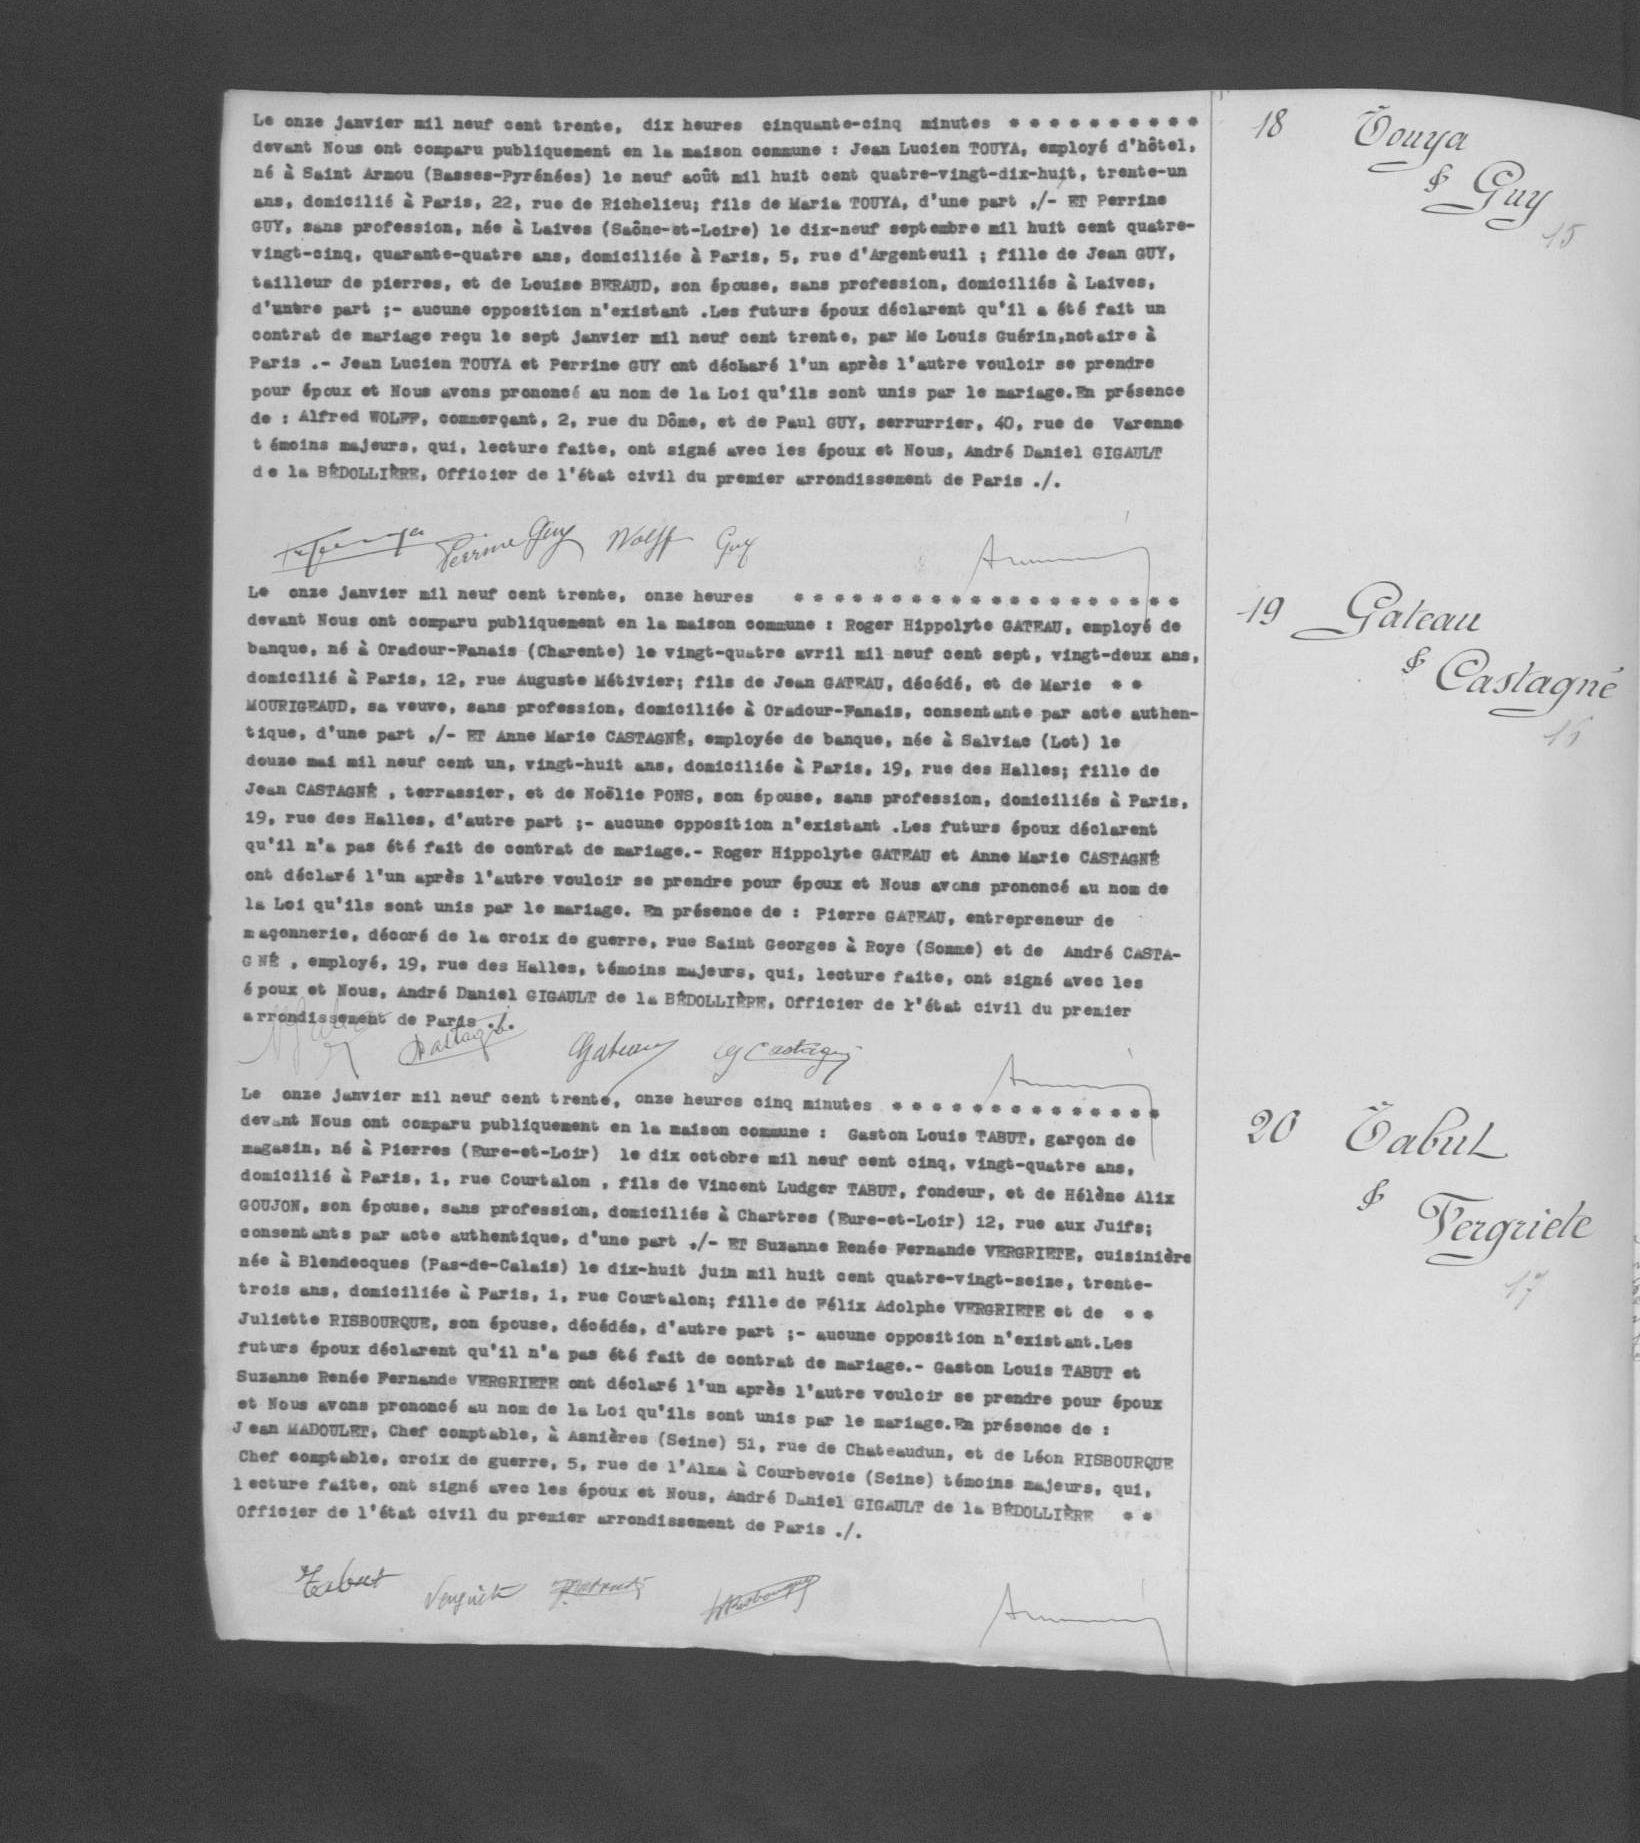Le onze janvier mil neuf cent trente, dix heures cinquante-cinq minutes ****
devant Nous ont comparu publiquement en la maison commune: Jean Lucien TOUYA, employé d'hôtel,
né à Saint Armou (Basses-Pyrénées) le neuf août mil huit cent quatre-vingt-dix-huit, trente-un
ans, domicilié à Paris, 22, rue de Richelieu; fils de Marie Touya, d'une part ./-ET Perrine
GUY, sans profession, née à Laives (Saône-et-Loire) le dix-neuf septembre mil huit cent quatre-
vingt-cinq, quarante-quatre ans, domiciliée à Paris, 5, rue d'Argenteuil; fille de Jean GUY,
tailleur de pierres, et de Louise BRRAUD, son épouse, sans profession, domiciliés à Laives,
d'autre part ;-aucune opposition n'existant. Les futurs époux déclarent qu'il a été fait un
contrat de mariage reçu le sept janvier mil neuf cent trente, par Mr Louis Guérin, notaire à
Paris .-Jean Lucien TOUYA et Perrine GUY ont déclaré l'un après l'autre vouloir se prendre
pour époux et Nous avons prononcé au nom de la Loi qu'ils sont unis par le mariage. En présence
de: Alfred WOLFF, commerçant, 2, rue du Dôme, et de Paul GUY, serrurrier, 40, rue de Varenne
témoins majeurs, qui, lecture faite, ont signé avec les époux et nous, André Daniel GIGAULT
de la BEDOLLIERE, Officier de l'état civil du premier arrondissement de Paris ./.
Le onze janvier mil neuf cent trente, onze heures ****
devant Nous ont comparu publiquement en la maison commune: Roger Hippoyte GATEAU, employé de
banque, né à Oradour-Fanais (Charente) le vingt-quatre avril mil neuf cent sept, vingt-deux ans,
domicilié à Paris, 12, rue Auguste Métivier; fils de Jean GATEAU, décédé, et de Marie * *
MOURIGEAUD, sa veuve, sans profession, domiciliée à Oradour-Fanais, consentante par acte authen
tique, d'une part ./-ET Anne Marie CASTAGNE, employée de banque, née à Salviac (Lot) le
douze mai mil neuf cent un, vingt-huit ans, domiciliée à Paris, 19, rue des Halles; fille de
Jean CASTAGNE, terrassier, et de Noëllie PONS, son épouse, sans profession, domiciliés à Paris,
19, rue des Halles, d'autre part ;-aucune opposition n'existant. Les futurs époux déclarent
qu'il n'a pas été fait de contrat de mariage .-Roger Hippolyte GATEAU et Anne Marie CASTAGNE
ont déclaré l'un après l'autre vouloir se prendre pour époux et Nous avons prononcé au nom de
la Loi qu'ils sont unis par le mariage. En présence de: Pierre GATEAU, entepreneur de
maçonnerie, décoré de la croix de guerre, rue Saint Georges à Roye (Somme) et de André CASTA-
GNE, employé, 19, rue des Halles, témoins majeurs qui, lecture faite, ont signé avec les
époux et Nous, André Daniel GIGAULT de la BEDOLLIERE, Officier de l'état civil du premier
arrondissement de Paris ./.
Le onze janvier mil neuf cent trente, onze heures cinq minutes ****
devant Nous ont ont comparu publiquement en la maison commune: Gaston Louis TABUT, garçon de
magasin, né à Pierres (Eure-et-Loir) le dix octobre mil neuf cent cinq, vingt-quatre ans,
domicilié à Paris, 1, rue Courtalon, fils de Vincent Ludger TABUT, fondeur, et de Hélène Alix
GOUJON, son épouse, sans profession, domiciliée à Chartres (Eure-et-Loir), 12, rue aux Juifs;
consentants par acte authentique, d'une part ./-ET Suzanne Renée Ferande VERGRIETE, cuisinière
née à Blendecques (Pas-de-Calais) le dix-huit juin mil huit cent quatre-vingt-seize, trente-
trois ans, domiciliée à Paris, 1, rue Courtalon; fille de Félix Adolphe VERGRIETE et de * *
Juliette RISBOURQUE, son épouse, décédés, d'autre part ;-aucune opposition n'existant. Les
futurs époux déclarent qu'il n'a pas été fait de contrat de mariage .-Gaston Louis TABUT et
Suzanne Renée Fernande VERGRIETE ont déclaré l'un après l'autre vouloir se prendre pour époux
et Nous avons prononcé au nom de la Loi qu'ils sont unis par le mariage. En présence de:
Jean MADOULET, Chef comptable, à Asnières (Seine) 51, rue de Chateaudun, et de Léon RISBOURQUE
Chef comptable, croix de guerre, 5, rue de l'Alsa à Courbevoie (Seine) témoins majeurs, qui,
lecture faite, ont signé avec les époux et Nous, André Daniel GIGAULT de la BEDOLLIERE * *
Officier de l'état civil du premier arrondissement de Paris ./.
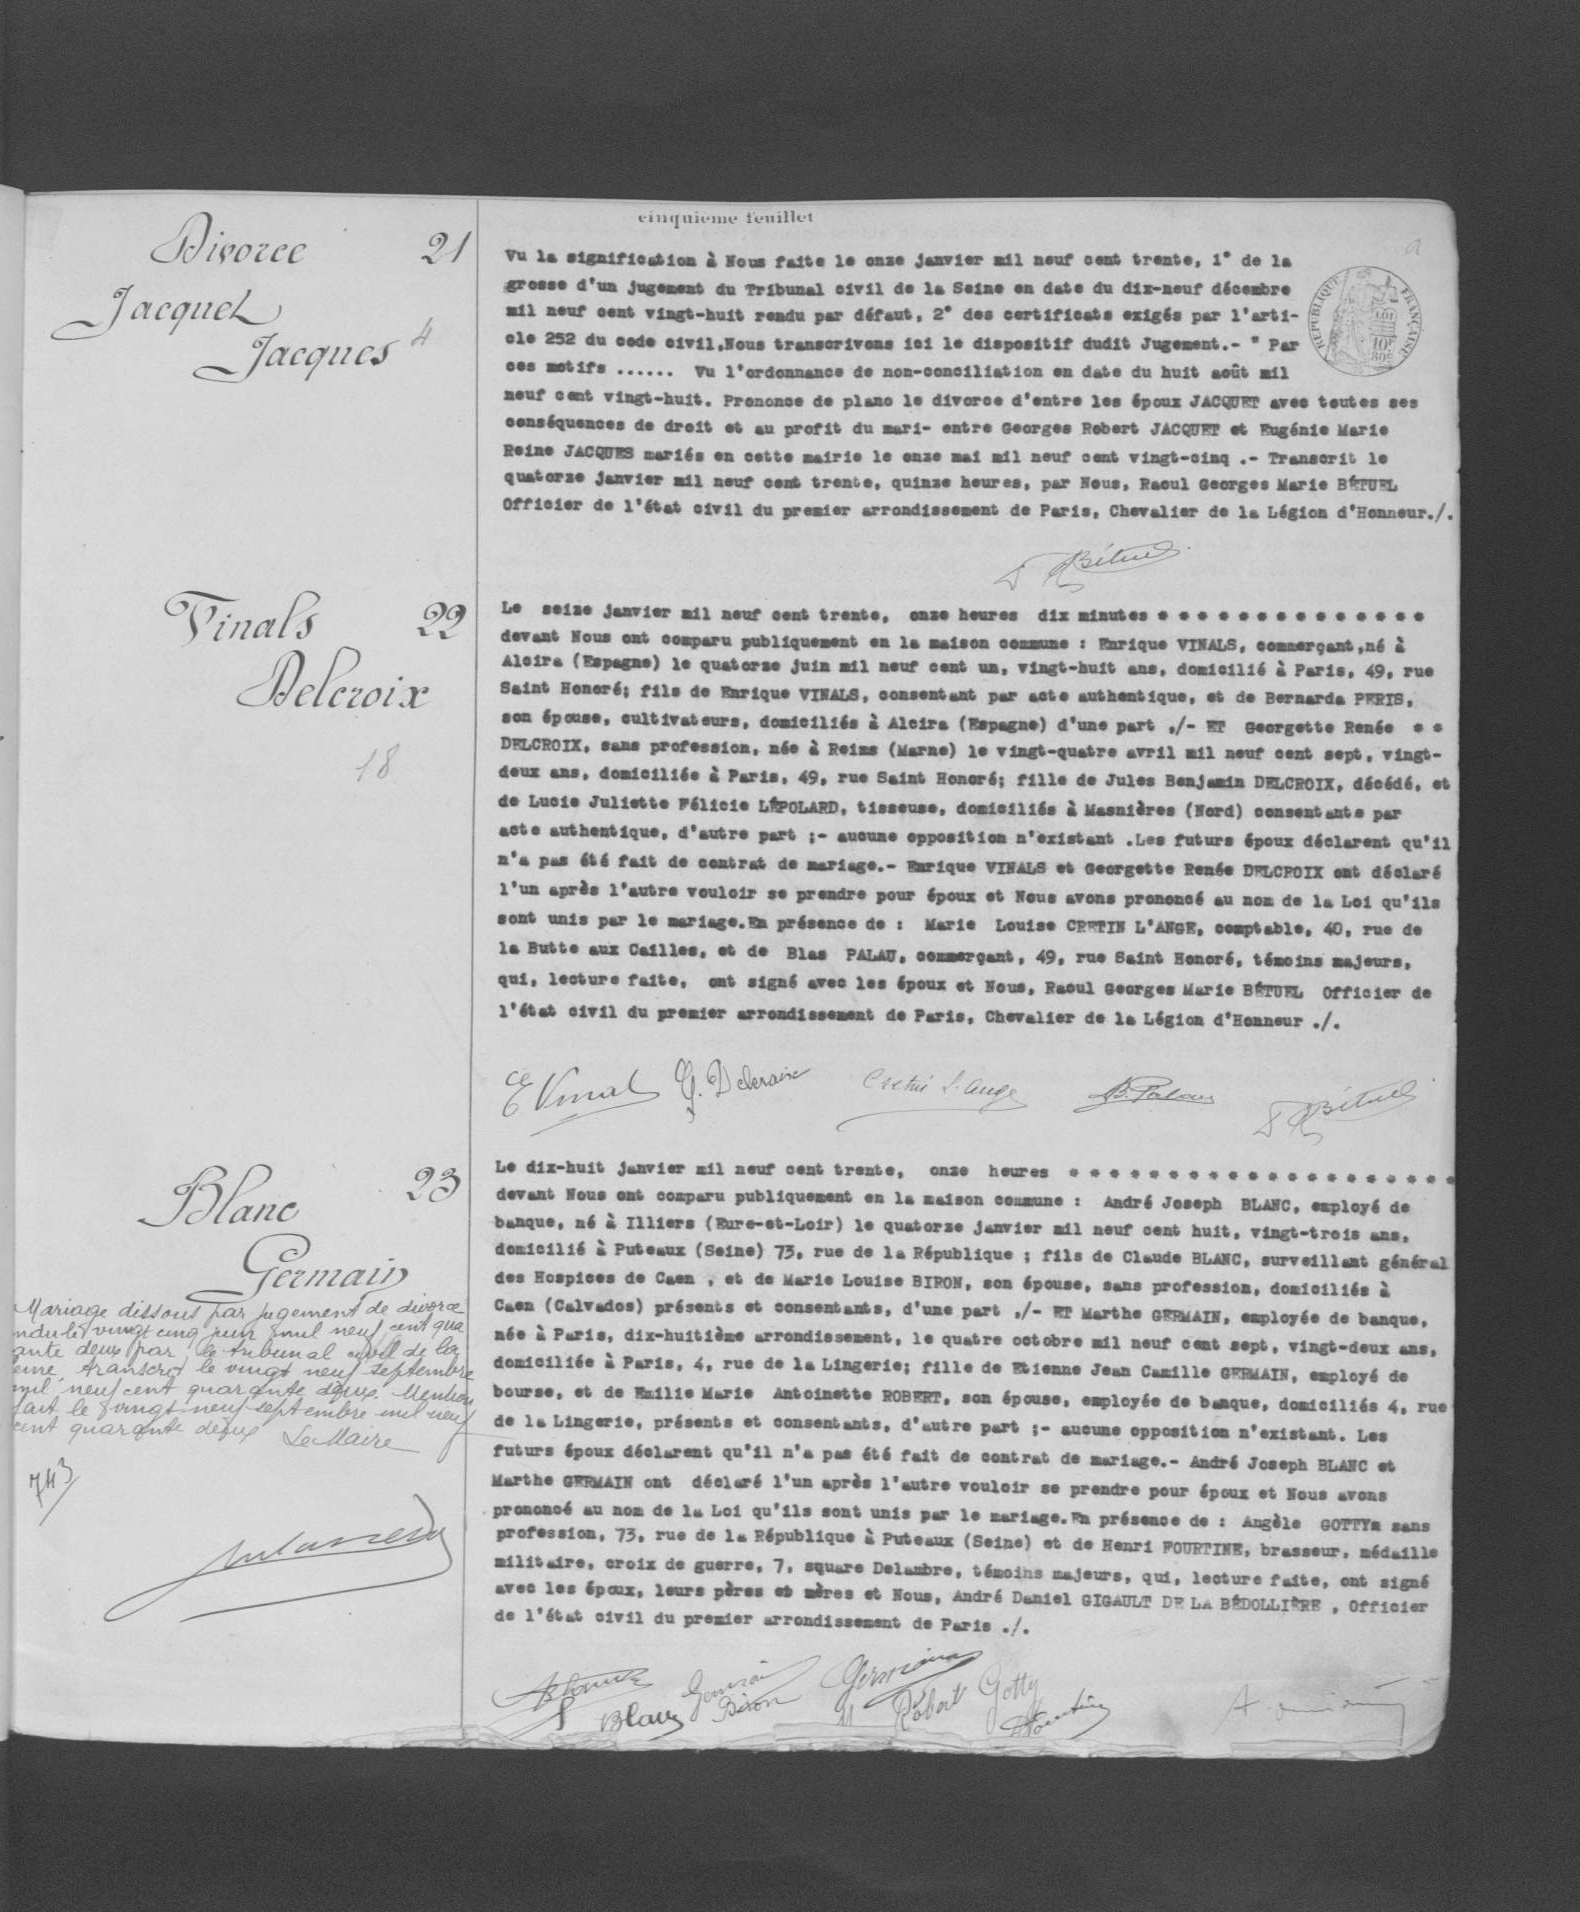Vu la signification à Nous faite le onze janvier mil neuf cent trente, 1° de la
grosse d'un jugement du Tribunal civil de la Seine en date du dix-neuf décembre
mil neuf cent vingt-huit rendu par défaut, 2° des certificats exigés par l'arti-
cle 252 du code civil, Nous transcrivons ici le dispositif dudit Jugement .-" Par
ces motifs ..... Vu l'ordonnance de non-conciliation en date du huit août mil
neuf cent vingt-huit. Prononce de plano le divorce d'entre les époux JACQUET avec toutes ses
conséquences de droit et au profit du mari-entre Georges Robert JACQUET et Eugénie Marie
Reine JACQUES mariés en cette mairie le onze mai mil neuf cent vingt-cinq .-Transcrit le
quatorze janvier mil neuf cent trente, quinze heures, par Nous, Raoul Georges Marie BETHUEL
Officier de l'état civil du premier arrondissement de Paris, Chevalier de la Légion d'Honneur ./.
Le seize janvier mil neuf cent trente, onze heures dix minutes ****
devant Nous ont comparu publiquement en la maison commune: Enrique VINALS, commerçant, né à
Alcira (Espagne) le quatorze juin mil neuf cent un, vingt-huit ans, domicilié à Paris, 49, rue
Saint Honoré; fils de Enrique VINALS, consentat par acte authentique, et de Bernarda PERIS,
son épouse, cultivateurs, domiciliés à Alcira (Espagne) d'une part ./-ET Georgette Renée * *
DELCROIX, sans profession, née à Reims (Marne) le vingt-quatre avril mil neuf cent sept, vingt-
deux ans, domiciliée à Paris, 49, rue Saint Honoré; fille de Jules Benjamin DELCROIX, décédé, et
de Lucie Juliette Félicie LEPOLARD, tisseuse, domiciliée à Masnières (Nord) consentants par
acte authentique, d'autre part ;-aucune opposition n'existant. Les futurs époux déclarent qu'il
n'a pas été fait de contrat de mariage .-Enrique VINALS et Georgette Renée DELCROIX ont déclaré
l'un après l'autre vouloir se prendre pour époux et Nous avons prononcé au nom de la Loi qu'ils
sont unis par le mariage. En présence de: Marie Louise CRETIN L'ANGE, comptable, 40, rue de
la Butte aux Cailles, et de Blas PALAU, commerçant, 49, rue Saint Honoré, témoins majeurs,
qui, lecture faite, ont signé avec les époux et Nous, Raoul Georges Marie BETUEL Officier de
l'état civil du premier arrondissement de Paris, Chevalier le la Légion d'Honneur ./.
Le dix-huit janvier mil neuf cent trente, onze heures ****
devant Nous ont comparu publiquement en la maison commune: André Joseph BLANC, employé de
banque, né à Illiers (Eure-et-Loir) le quatorze janvier mil neuf cent huit, vingt trois ans,
domicilié à Pluteaux (Seine) 73, rue de la République; fils de Claude BLANC, surveillant général
des Hospices de Caen, et de Marie Louise BIRON, son épouse, sans profession, domiciliés à
Caen (Calvados) présents et consentants, d'une part ./-ET Marthe GERMAIN, employée de banque,
née à Paris, dix-huitième arrondissement, le quatre octobre mil neuf cent sept, vingt-deux ans,
domiciliée à Paris, 4, rue de la Lingerie; fille de Etienne Jean Camille GERMAIN, employé de
bourse, et de Emilie Marie Antoinette ROBERT, son épouse, employée de banque, domiciliés 4, rue
de la Lingerie, présents et consentants, d'autre part ;-aucune opposition n'existant. Les
futurs époux déclarent qu'il n'a pas été fait de contrat de mariage .-André Joseph BLANC et
Marthe GERMAIN ont déclaré l'un après l'autre vouloir se prendre pour époux et Nous avons
prononcé au nom de la Loi qu'ils sont unis par le mariage. En présence de: Angèle GOTTY, sans
profession, 73, rue de la République à Puteaux (Seine) et de Henri FOURTINE, brasseur, médaille
militaire, croix de guerre, 7, square Delambre, témoins majeurs, qui, lecture faite, ont signé
avec les époux, leurs pères et mères et Nous, André Daniel GIGAULT de la BEDOLLIERE, Officier
de l'état civil du premier arrondissement de Paris.